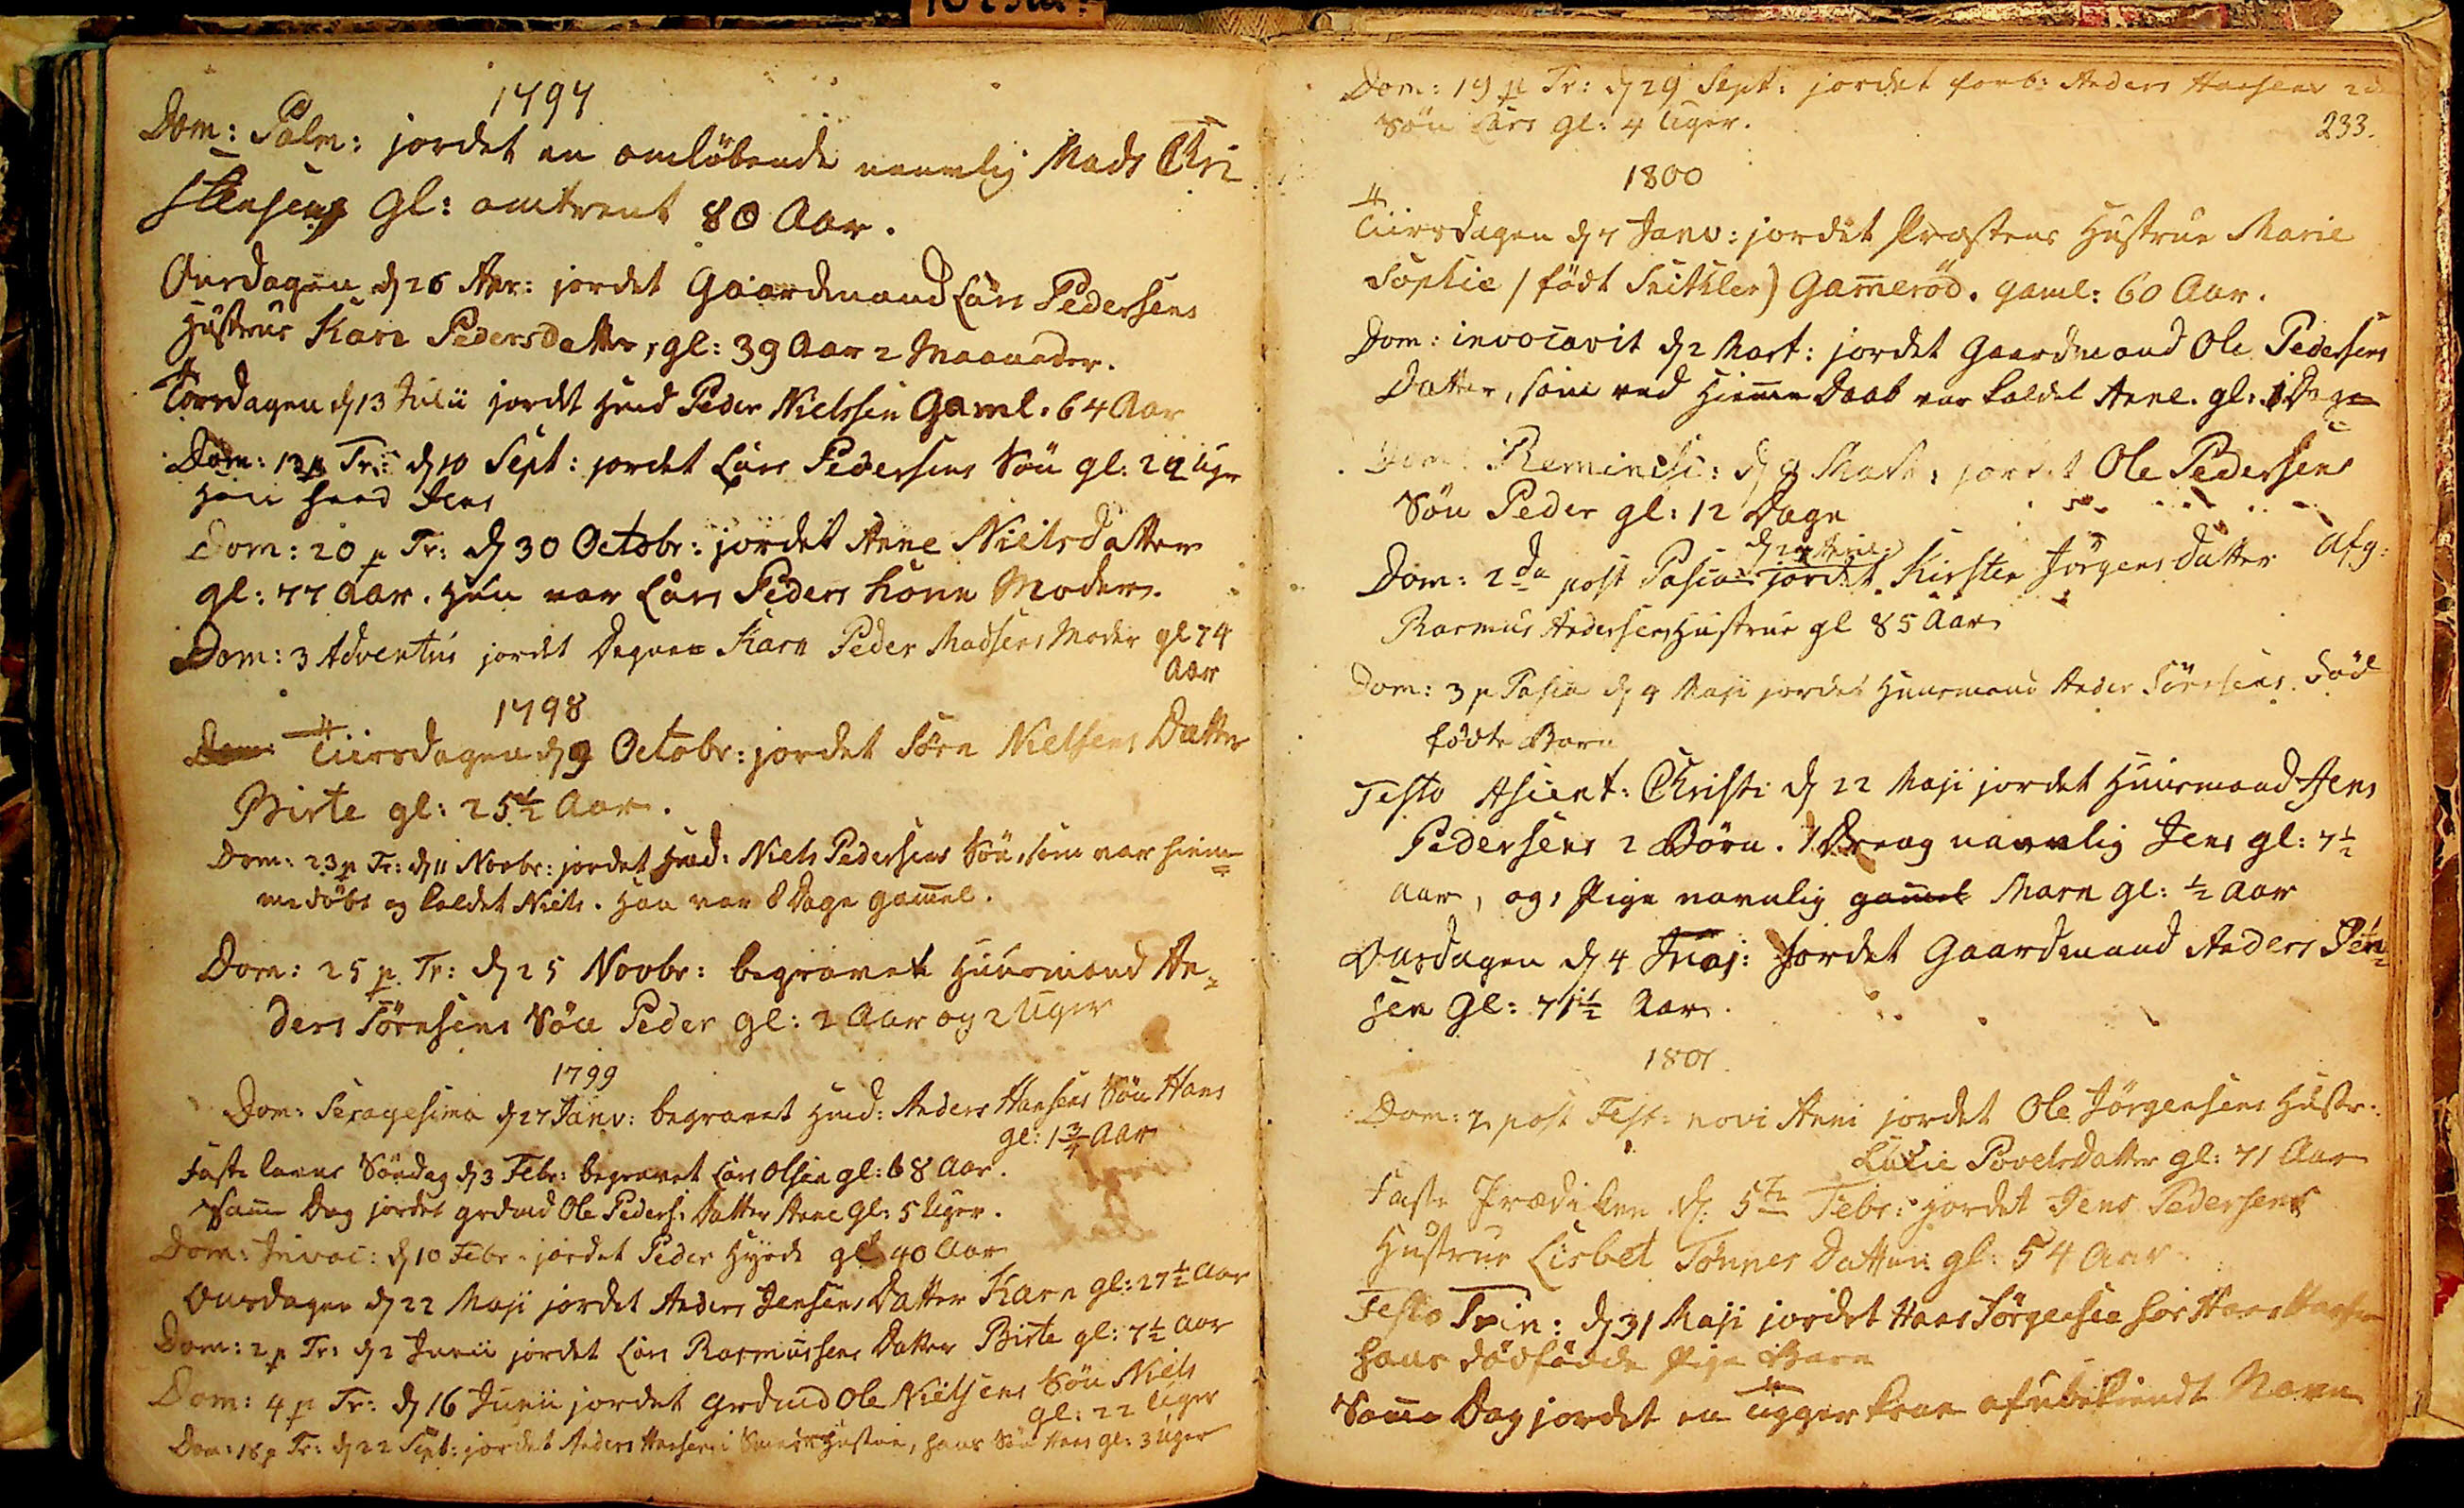1797
Dom: Palm: jordet en omløbende navnlig Mads Chri¬
stensen gl: omtrent 80 Aar.
Onsdagen d 26 Apr: jordet Gaardmand Lars Pedersens
Hustrue Karn Pedersdatter, gl: 39 Aar 2 Maaneder.
Torsdagen d 13 Julii jordet Hmd Peder Nielssen Gaml: 64 Aar
Dom: 13 p Tr: d 10 Sept: jordet Lars Pedersens Søn gl 22 Uger
Han heed Jens
Dom: 20 p Tr: d 30 Octobr: jordet Anne Nielsdatter
gl: 77 Aar. Hun var Lars Peders Kone Moder.
Dom: 3 Adventus jordet Degnen Karn Peder Madsens Moder gl 74
Aar
1798
Den Tiirsdagen d 9 Octobr: jordet Sørn Nielsens Datter
Birte gl: 25½ Aar.
Dom: 23 p tr: d 11 Novbr: jordet Hmd: Niels Pedersens Søn, som var hiem¬
medøbt og kaldet Niels. Han var 8 Dage gammel.
Dom: 25 p Tr: d 25 Novbr: begravet Huusmand An¬
ders Sørnsens Søn Peder gl: 2 Aar og 2 Uger
1799
Dom: Sexagesima d 27 Janu: begravet Hmd: Anders Hansen Søn Hans
gl: 1 3/4 Aar
Fastelavns Søndag d 3 Febr: begravet Lars Olsen gl: 68 Aar.
Same Dag jordet Grdmd Ole Peders: Datter Anne gl: 5 Uger.
Dom: Invoc: d 10 Febr: jordet Peder Hyrde gl 40 Aar
Onsdagen d 22 Maji jordet Anders Jensens Datter Karn gl: 27½ Aar
Dom: 2 p Tr: d 2 Junii jordet Lars Rasmussens Datter Birte gl: 7½ Aar
Dom: 4 p Tr: d 16 Junii jordet Grdmd Ole Nielsens Søn Niels
gl: 22 Uger
Dom: 18 p Tr: d 22 Sept: jordet Anders Hansen i Smede Hust##, hans Søn Hans: 3 Uger
233.
Dom: 19 p Tr: d 29 Sept: jordet forb: Anders Hansens 2den
Søn Lars gl:4 Uger.
1800
Tiirsdagen d 7 Janu: jordet Præstens Hustrue Marie
Sophie (født Snithler) Gammerød. Gamel: 60 Aar.
Dom: invocavit d 2 Mart: jordet Gaardmand Ole Pedersens
Datter, som ved Hieme Daab var kaldet Anne. gl: 1 dage
Dom: Reminisc: d 9 Mart: jordet Ole Pedersens
Søn Peder gl: 12 dage
d 24 April
Dom: 2da post Pasca jordet Kirsten Jørgensdatter Afg:
Rasmus Andersens Hustrue gl: 85 Aar
Dom: 3 p Pasca d 4 Maji jordet Huusmand Anders Sørnsens død
fødte Barn.
Festo Ascent: Christi d 22 Maji jordet Huusmand Jens
Pedersens 2 Børn. 1 Dreng navnlig Jens gl: 7½
Aar, og 1 Pige navnlig gammel Marn gl: ½ Aar
Onsdagen d 4 Juni: jordet Gaardmand Anders Jen¬
sen Gl: 71½ Aar.
1801
Dom: 2 post Fest: novi Anni jordet Ole Jørgensens Hustr:
Lusie Povelsdatter gl: 71 Aar
Faste Prædiken d: 5te Febr: jordet Jens Pedersens
Hustrue Lisbet Tønnes Datter gl: 54 Aar
Festo Trin: d 31 Maji jordet Hans Jørgensen hos Hans Hansen
hans dødfødde Pige Barn
Same Dag jordet en Tigger kone af ubekiendt Navn